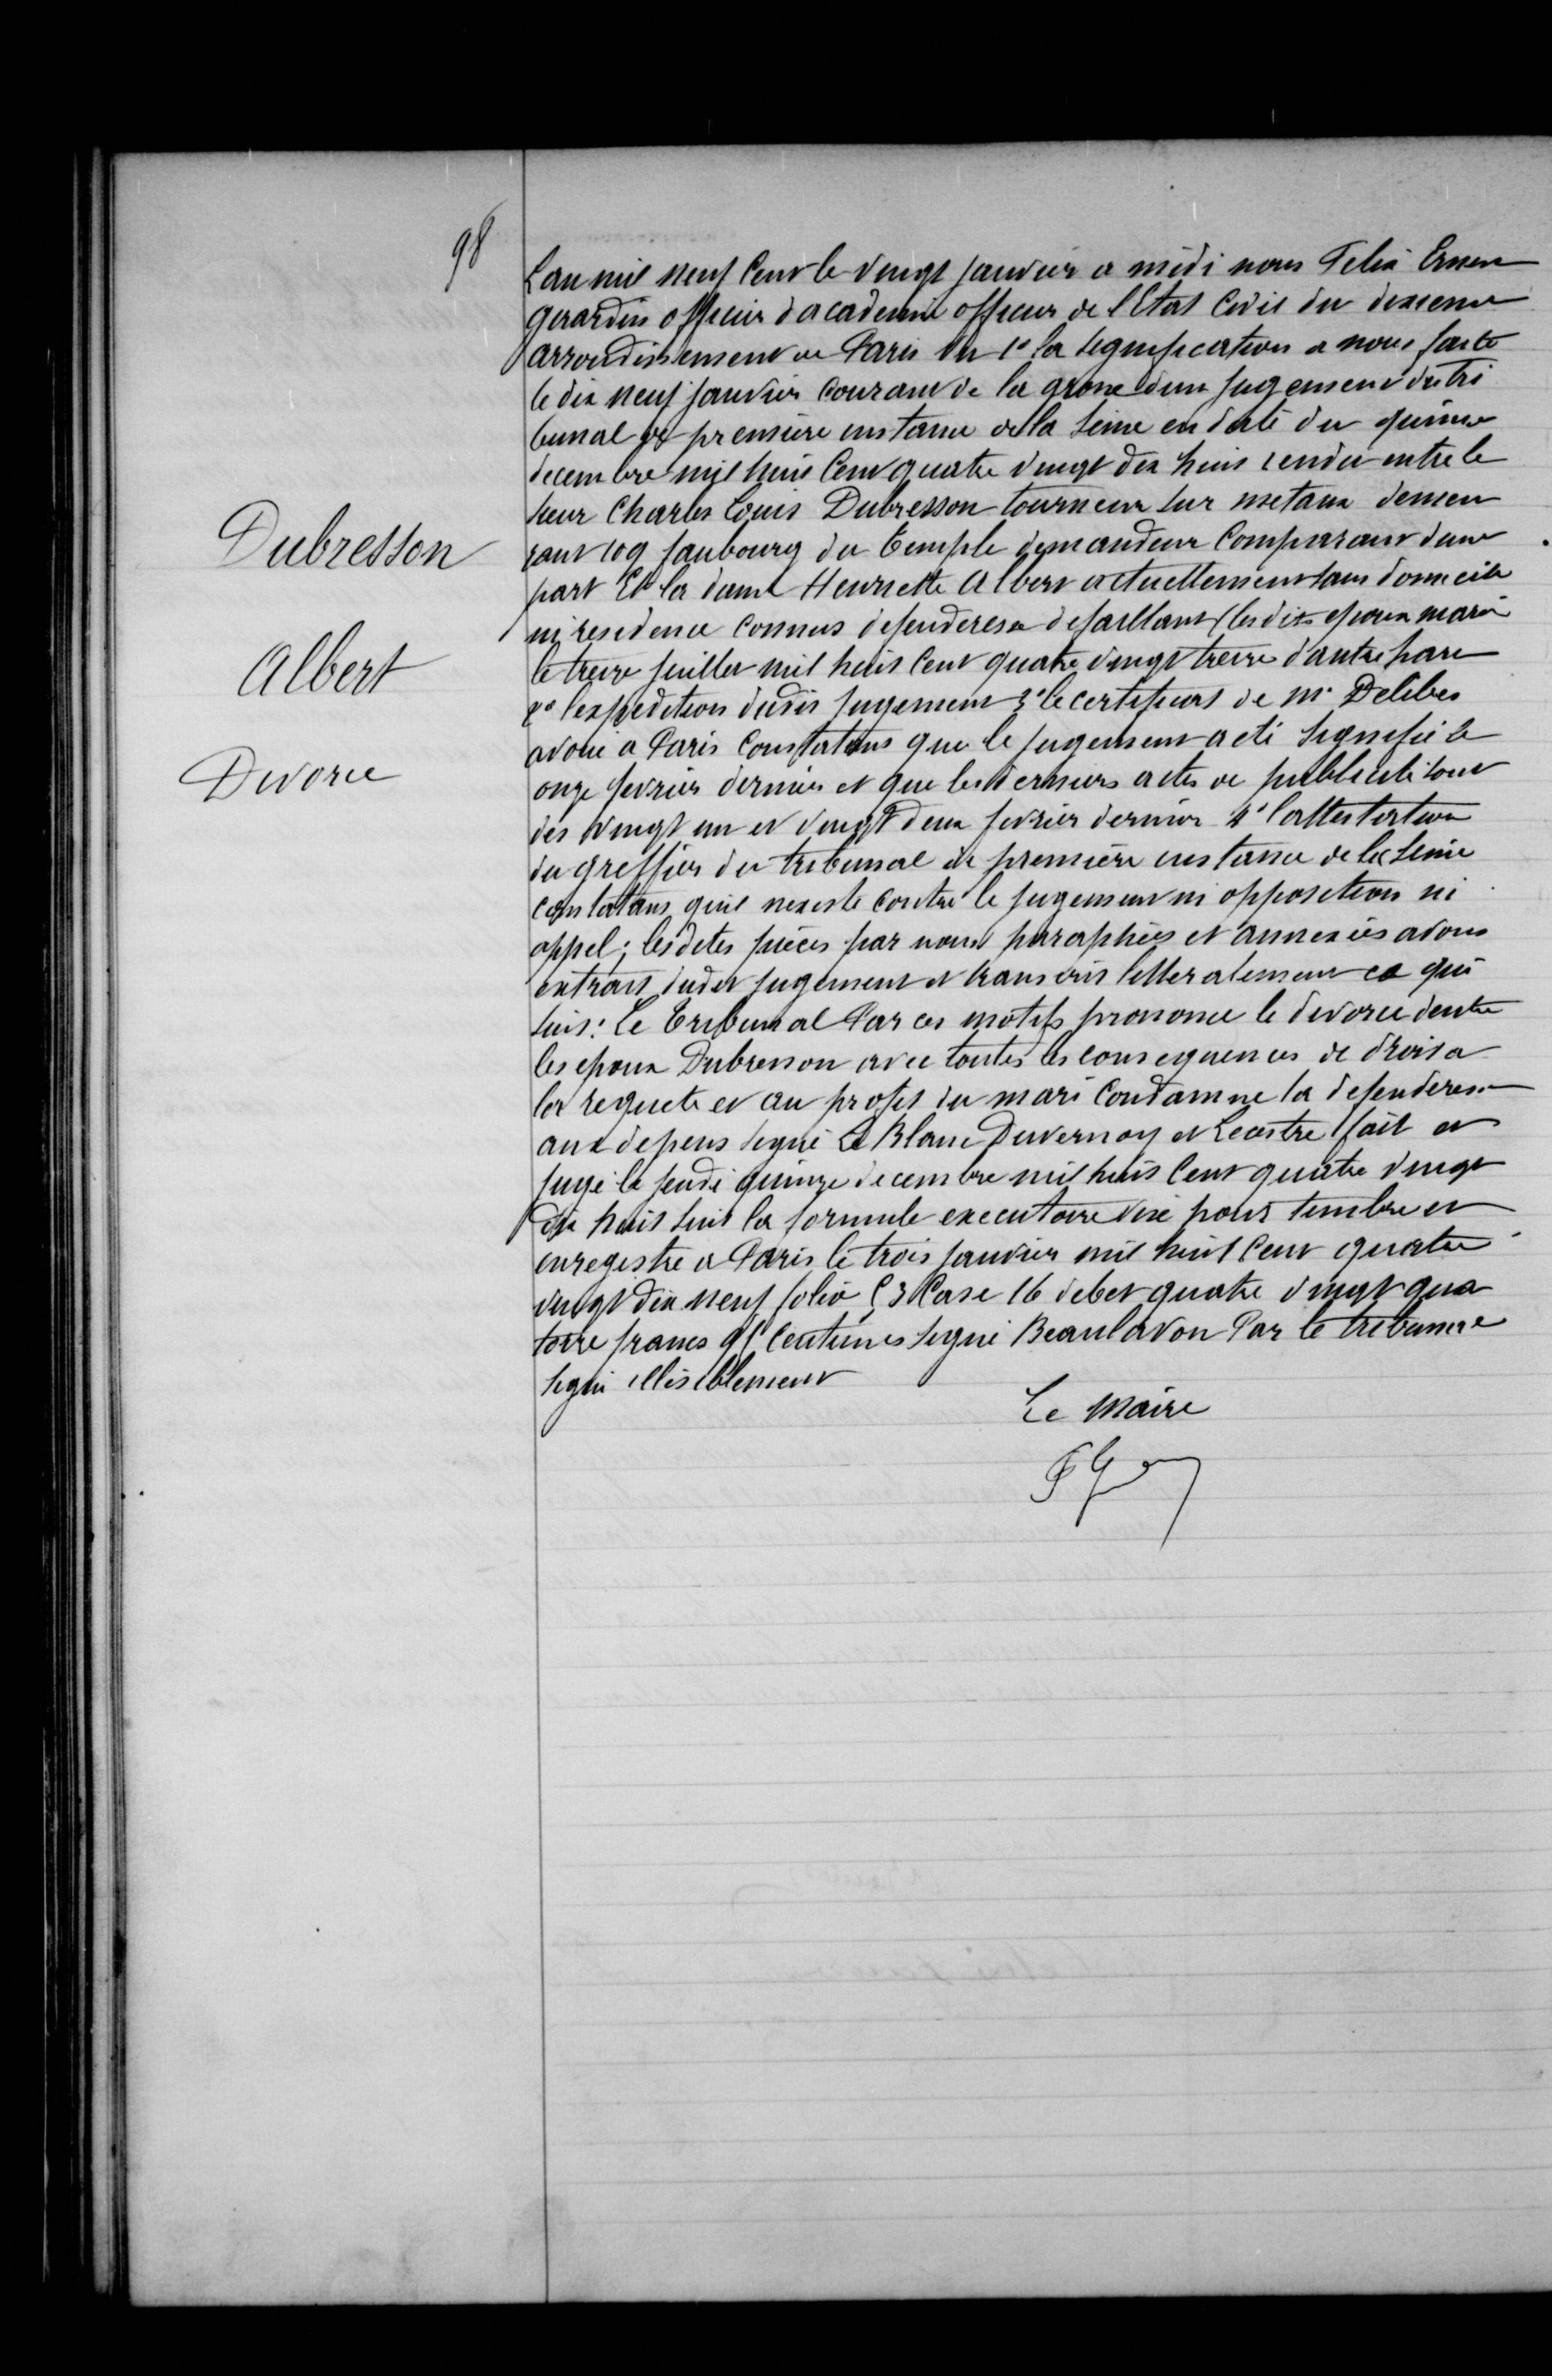Dubresson
Albert
Divorce
L'an mil neuf cent le vingt Janvier a midi nous Felix Ernest
Firardin officier d'academie officier de l'Etat Civil du dixieme
arrondissement de Paris vu 1° la signification a nous faite
le dix neuf janvier courant de la grosse d'un jugement du tri
bunal de première instance de la Seine en date du quinze
decembre mil huit cent quatre vingt dix huit rendu entre le
Sieur Charles louis Dubresson tourneur sur metaux demeu
rant 109 faubourg du temple demandeur comparant d'une
part Et la dame Heurette Albert actuellement sans domicile
ni résidence connus defenderesse defaillant (les dix epoux marié
le treize juillet mil huit cent quatre vingt treize d'autre part
2° l'expedition dudis Jugement 3° le certificat de M° Delibes
avoué à Paris Constatans que le jugement acté signifié le
onze fevrier dernier et que les derniers actes de publication
des vingt un et vingt deux fevrier dernier 4° l'attestation
du greffier du tribunal de première instance de lecture
constantans qu'il nexiste contre le jugement ni opposition ni
appel; les dites pièces par nous paraphées et annexées avons
extrais dudit Jugement et transcris litteralement ce qui
suis: Le tribunal Par ces motifs prononce le divorce dont
les epoux Dubresson avec toutes les consequences de droits a
la requete et aux profis du mari Condamne la defenderesse
aux depens signé Le Blanc Duvernay et Leantre fait et
Jugé le jeudi quinze decembre mil huis cent quatre vingt
dix huit suit mil la formule executoire visé pour timbre et
enregistre a Paris le trois janvier mil huit cent quatre
vingt dix neuf folio 53 Case 16 debet quatre vingt qua
torze francs 95 centimes signé Beaulavon Par le tribunal
signé illisiblement
Le Maire
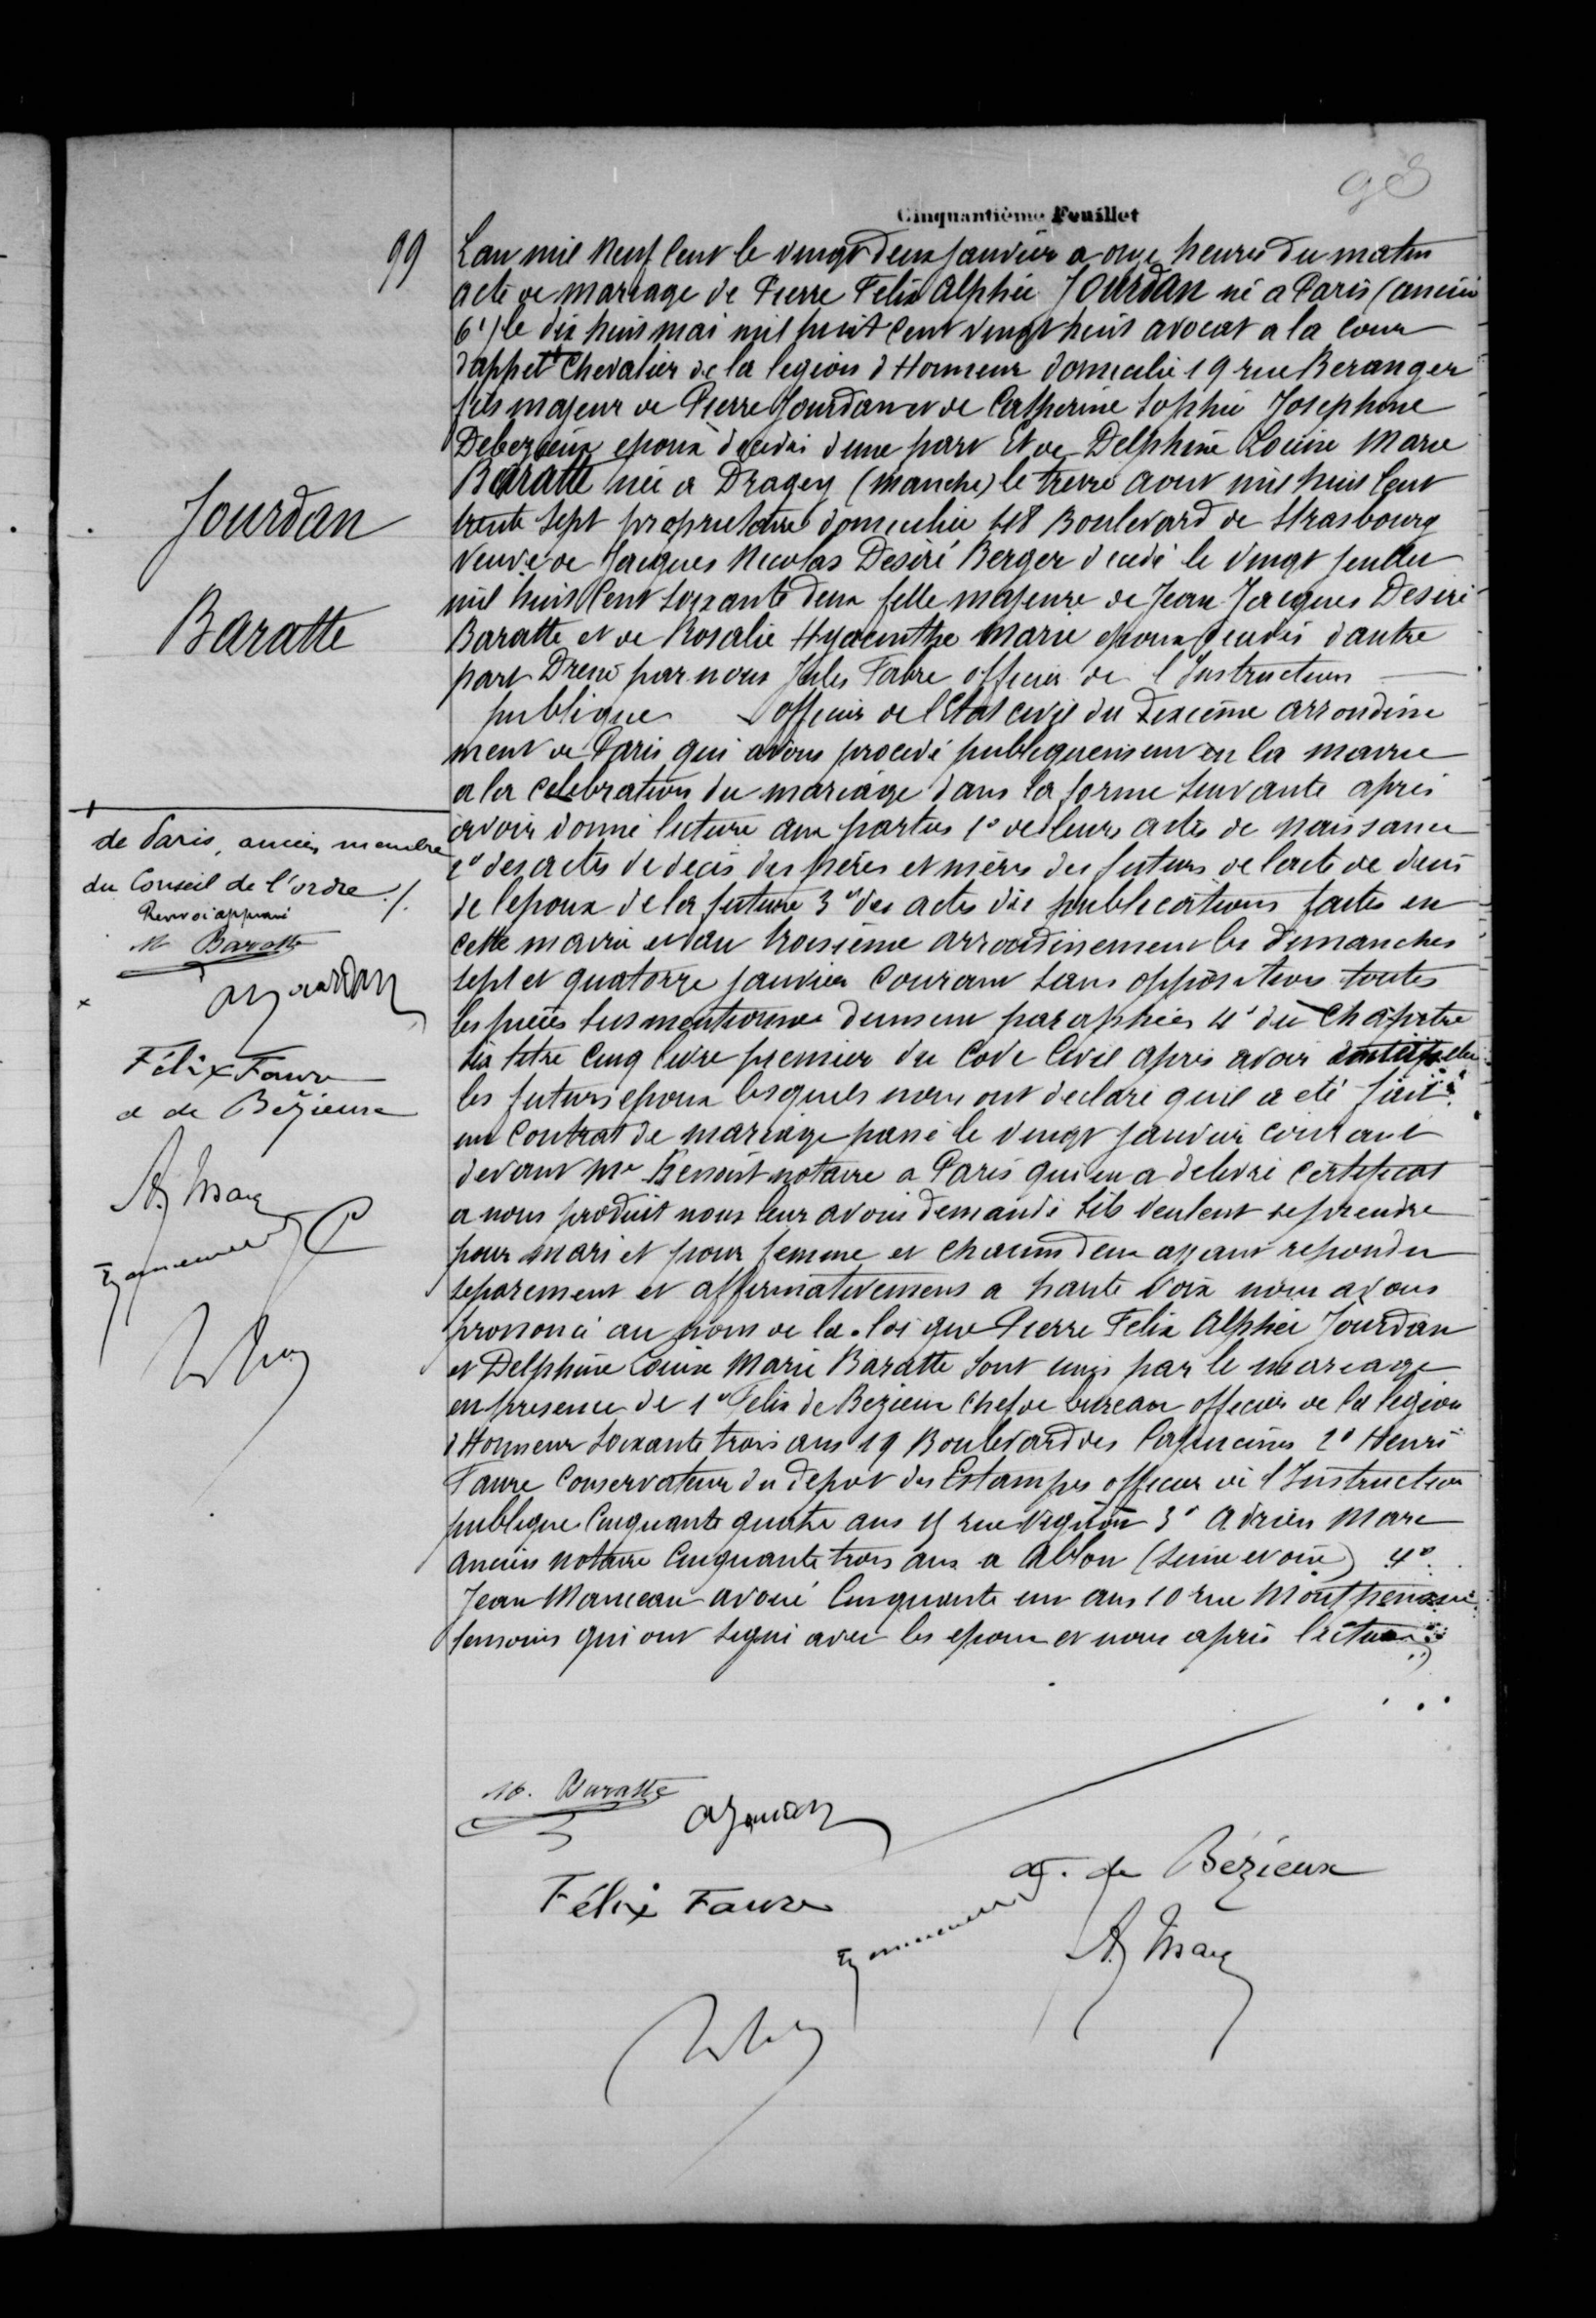Jourdan
Baratte
L'an mil neuf cent le vingt deux janvier a onze heure du matin
acte de mariage de Pierre Felix Alphée Jourdan né à Paris (ancien
6°) le dix juit mai mil huit cent vingt huit avocat a la cour
d'appel Chevalier de la legion d'Honneur domicilie 19 rue Beranger
fils majeur de Pierre Jourdan et de Catherine doppui Josephine
Deberieux epoux décédés d'une part Et de DElphin Locine Marie
Baratte née a Dragny (Manche) le treize aout mil huis cent
trente sept proprutoire dimanche 48 Boulevard de Strasbourg
Veuve de Jacques Nicolas Desiré Berger decedé le vingt fevrier
mil huis cent soixante deux fille majeure de Jean-Jacques Desire
Baratte et de Rosalie Hyaenthe Marie epoux decedés d'autre
part Dressé par nous Jules Fabre officier de l'Instruction -
publique. Officier de l'Etat Civil du dixième arrondisse
ment de Paris qui avons presenté publiquement en la mairie
a la celebration du mariage dans la forme suivante après
avoir donné lecture aux parties 1° de leurs actes de naissance
2° des actes de décès des pères et mères des futurs de l'acte de décès
de l'epoux de la future 3° des actes des publications faites en
cette mairie et au troisième arrondissement le dimanches
Sept et quatorze janvier courant sans oppositions toutes
les pièces susmentionnés dument paraphées 4° du Chapitre
six titre cinq livre premier du Code Civil après avoir interpellé
les futurs epoux lesquels nous ont declaré qu'il a été fait
un contrat de mariage passé le vingt janvier courant
devant Me Benoit notaire a Paris qui en a delivré certificat
a nous produit nous leur avons demandé sils veulent de prendre
pour mari et pour femme et chacun deux ayant repondu
separement et affirmativement a haute voix nous avons
prononcé au nom de la loi que Pierre Felix Alphé Jourdan
et Delphine Louise Marie Baratte sont unis par le mariage
en presence de 1° Felix de Bezieux Chef de bureau officier de la legion
d'Honneur Soixante trois ans 19 Boulevard des Capucines 2° Henri
Faure Conservateur du depot des Estampes officier de l'Instruction
publique, Cinquante quatre ans 25 rue Vignon 3° Adrien Marc
ancien notaire Cinquante trois ans a Ablon (Seine et Oise) 4°
Jean Monceau avoué Cinquante un ans 10 rue Montpensier
temoins qui ont signé avec les epoux et nous après lecture.